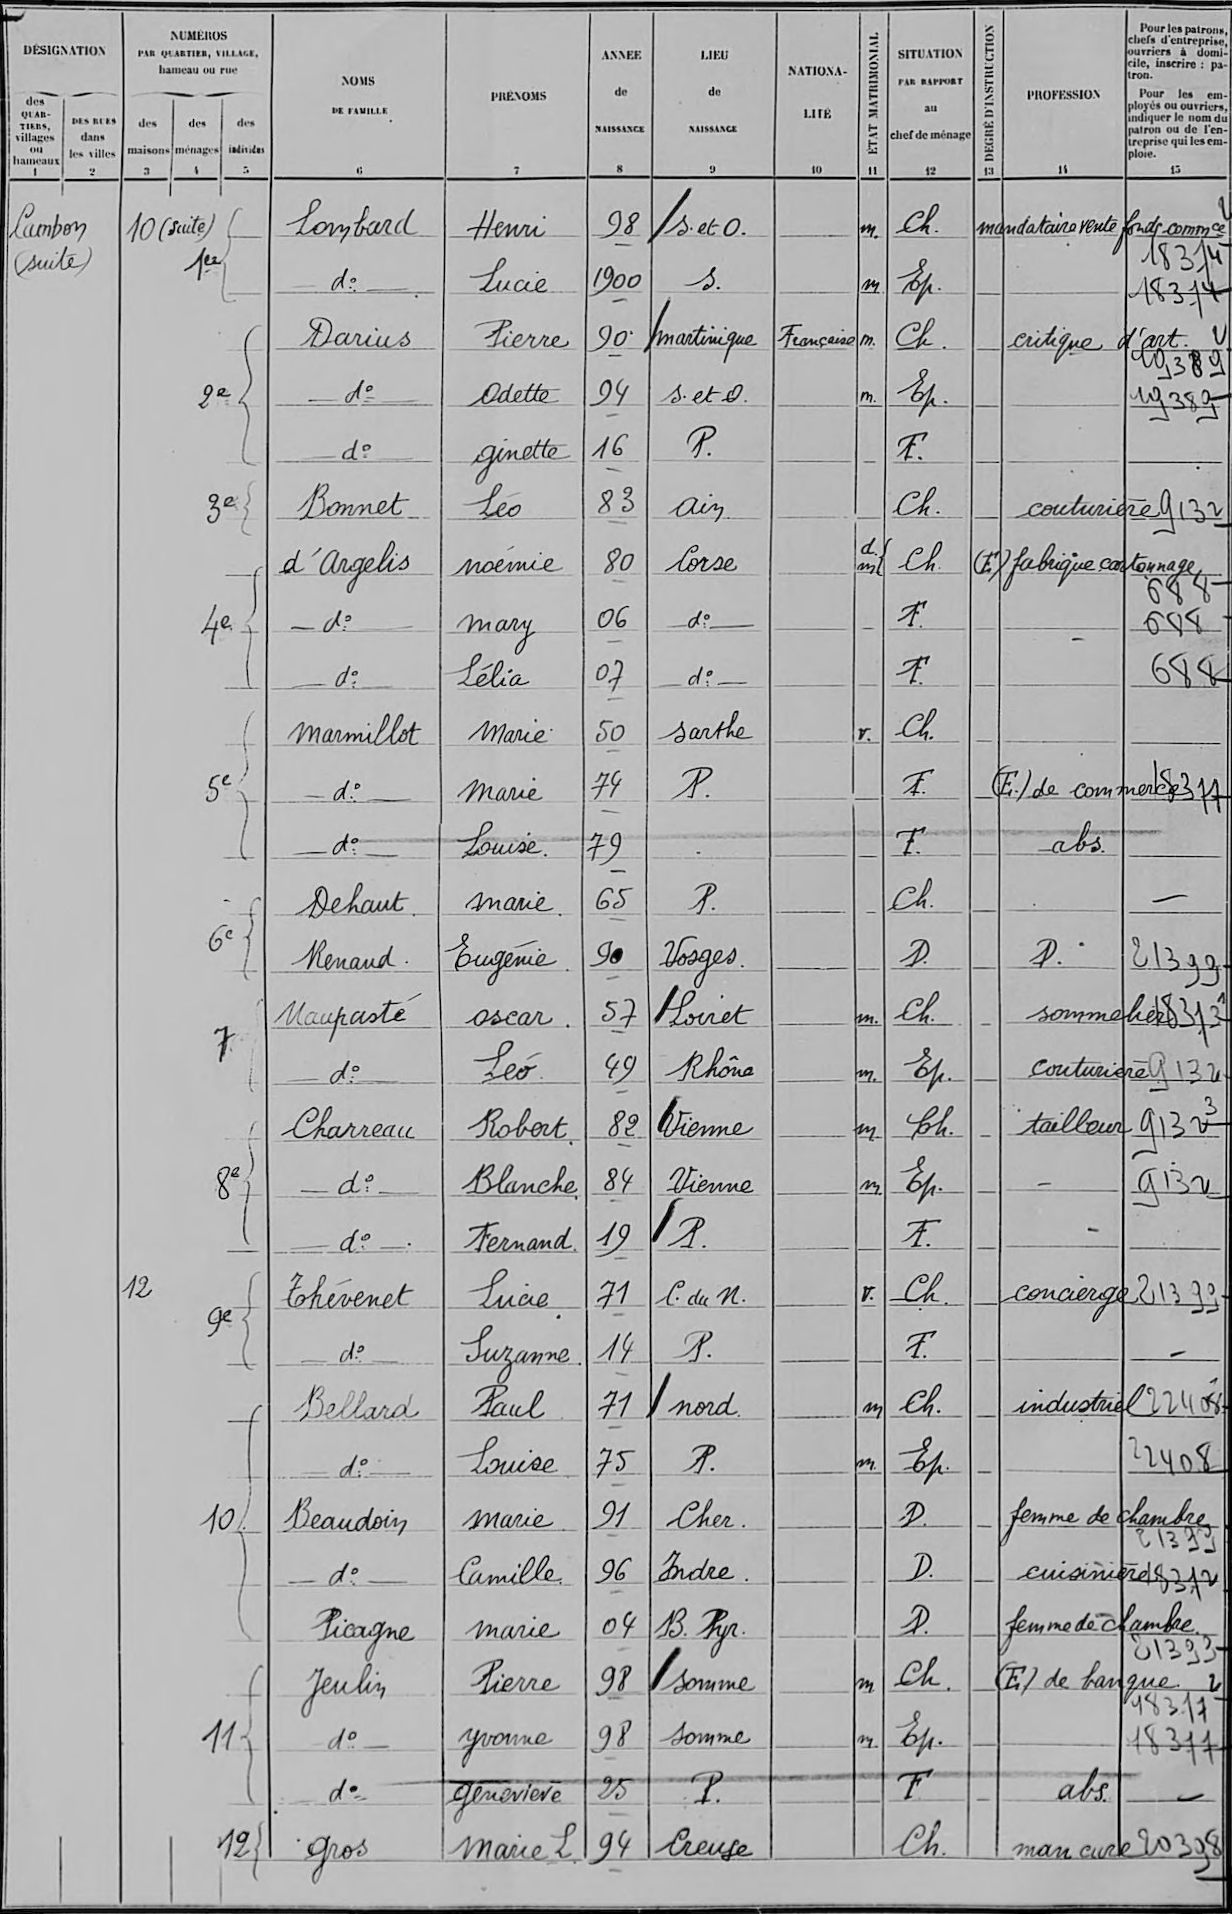Lombard/Henri/98/s et o /¤/m /Ch /¤/mandataire vente fonds commce/!18374
d°/Lucie/1900/s /¤/m /Ep /¤/¤/18374
Darius/Pierre/90/martinique/Française/m /Ch /¤/critique d'art/!19389
d°/Odette/94/s et o /¤/m /Ep /¤/¤/19389
d°/ginette/16/P /¤/¤/F /¤/¤/¤
Bonnet/Léo/83/Ain/¤/¤/Ch /¤/couturière/9132
d'Argelis/noémie/80/Corse/¤/m?d/Ch /¤/(E) fabrique cartonnage/!688
d°/mary/06/d°/¤/¤/F /¤/¤/688
d°/Lélia/07/d°/¤/¤/F /¤/¤/688
Marmillot/Marie/50/Sarthe/¤/v /Ch /¤/¤/¤
d°/Marie/74/P /¤/¤/F /¤/(E ) de commerce/18377
d°/Louise/79/¤/¤/¤/F /¤/abs/¤
Dehaut/Marie/65/P /¤/¤/Ch /¤/¤/¤
Renaud/Eugénie/90/Vosges/¤/¤/D /¤/D /21399
Maupasté/oscar/57/Loiret/¤/m /Ch /¤/sommelier/18373
d°/Léo/49/Rhône/¤/m /Ep /¤/couturière/9132
Charreau/Robert/82/Vienne/¤/m/Ch/¤/tailleur/9132
d°/Blanche/84/Vienne/¤/m /Ep /¤/¤/9132
d°/Fernand/19/P /¤/¤/F /¤/¤/¤
Thévenet/Lucie/71/C du N /¤/v /Ch /¤/concierge/21399
d°/Suzanne/14/P /¤/¤/F /¤/¤/¤
Bellard/Paul/71/nord/¤/m/Ch /¤/industriel/22408
d°/Louise/75/P /¤/m /Ep /¤/¤/22408
Beaudoin/Marie/91/Cher/¤/¤/P /¤/femme de chambre/!21399
d°/Camille/96/Indre/¤/¤/D /¤/cuisinière/18372
Picagne/marie/04/B Pyr /¤/¤/D /¤/femme de chambre/!21399
Jeulin/Pierre/98/Somme/¤/m/Ch /¤/(E) de banque/!18377
d°/Yvonne/98/Somme/¤/m/Ep /¤/¤/18377
d°/Geneviève/25/P /¤/¤/F/¤/abs /¤
Gros/Marie L/94/Creuse/¤/¤/Ch /¤/man cure/20398
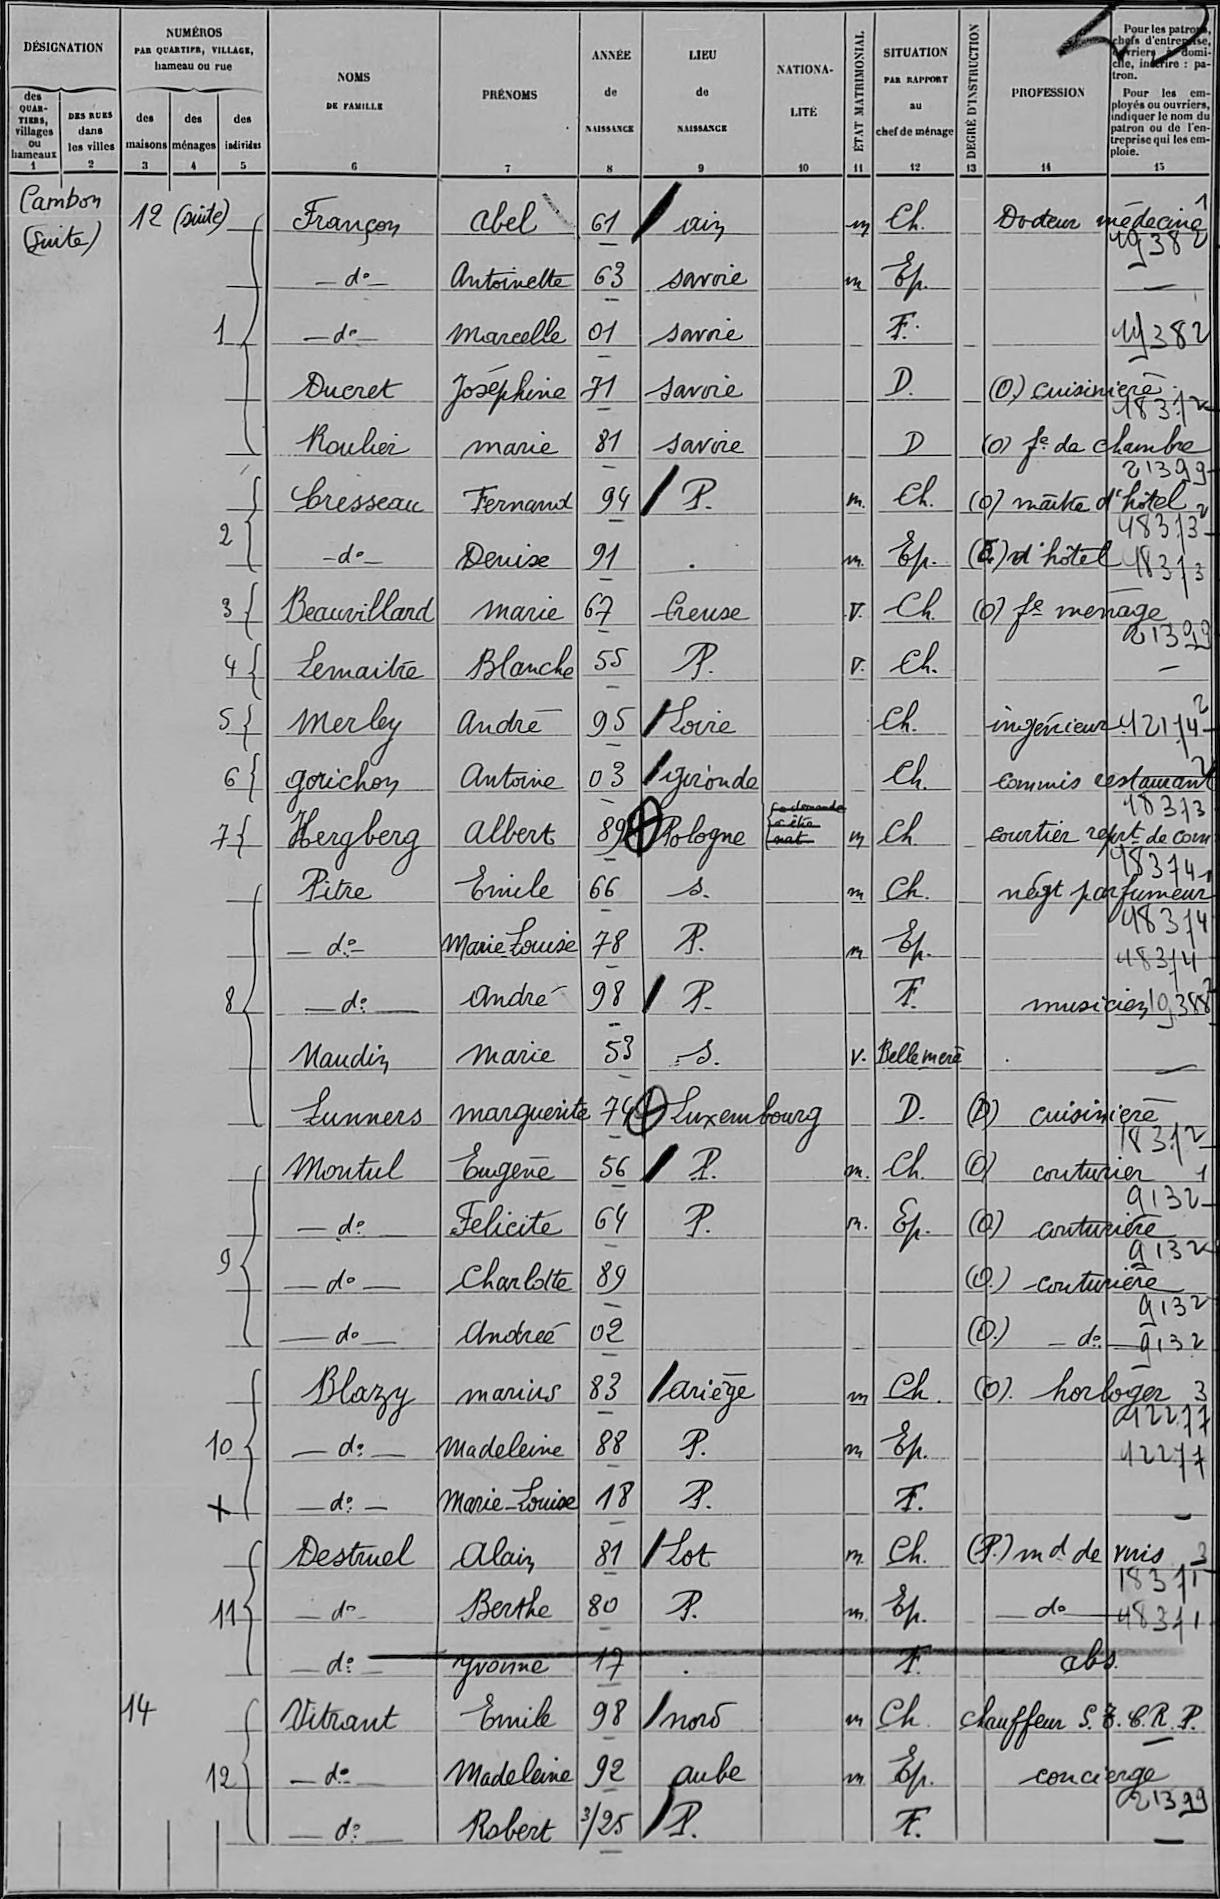Françon/Abel/61/ain/¤/m/Ch /¤/Docteur médecine/!19382
d°/Antoinette/63/Savoie/¤/m/Ep /¤/¤/¤
d°/marcelle/01/Savoie/¤/¤/F /¤/¤/19382
Ducret/Joséphine/71/Savoie/¤/¤/D /¤/(O) cuisinière/!18372
Roulier/marie/81/Savoie/¤/¤/D/¤/(O) fe de chambre/!21399
Cresseau/Fernand/94/P /¤/m /Ch /¤/(o) maître d'hôtel/!18373
d°/Denise/91/¤/¤/m /Ep /¤/(E) d'hôtel/18373
Beauvillard/marie/67/Creuse/¤/v /Ch/¤/(O) fe ménage/!21399
Lemaitre/Blanche/55/P /¤/v /Ch /¤/¤/¤
Merley/André/95/Loire/¤/¤/Ch /¤/ingénieur/12174
Gorichon/Antoine/03/Gironde/¤/¤/Ch /¤/commis restaurant/!18373
Hergberg/Albert/89/Pologne/¤/m/Ch/¤/courtier reprt de com/!18374
Pitre/Emile/66/S /¤/m/Ch /¤/négt parfumeur/!18374
d°/Marie Louise/78/P /¤/m/Ep /¤/¤/18374
d°/André/98/P /¤/¤/F /¤/musicien/19388
Naudin/Marie/53/S /¤/v /Belle mère/¤/¤/¤
Lunners/marguerite/74/Luxembourg/¤/¤/D /¤/(D) cuisinière/!18372
Montul/Eugène/56/P /¤/m /Ch /¤/(o) couturier/!9132
d°/Felicité/64/P /¤/m /Ep /¤/(O) couturière/!9132
d°/Charlotte/89/¤/¤/¤/¤/¤/(O) couturière/!9132
d°/Andrée/02/¤/¤/¤/¤/¤/(O) d°/9132
Blazy/marius/83/Ariège/¤/m/Ch /¤/(O) horloger/!12277
d°/Madeleine/88/P /¤/m /Ep /¤/¤/12277
d°/Marie-Louise/18/P /¤/¤/F /¤/¤/¤
Destruel/Alain/81/Lot/¤/m /Ch /¤/(P) md de vins/!18371
d°/Berthe/80/P /¤/m /Ep /¤/d°/18371
d°/Yvonne/17/¤/¤/¤/F /¤/abs/¤
Vitrant/Emile/98/nord/¤/m/Ch /¤/chauffeur/S T C R P
d°/Madeleine/92/aube/¤/m /Ep /¤/concierge/!21399
d°/Robert/3:25/P /¤/¤/F /¤/¤/¤